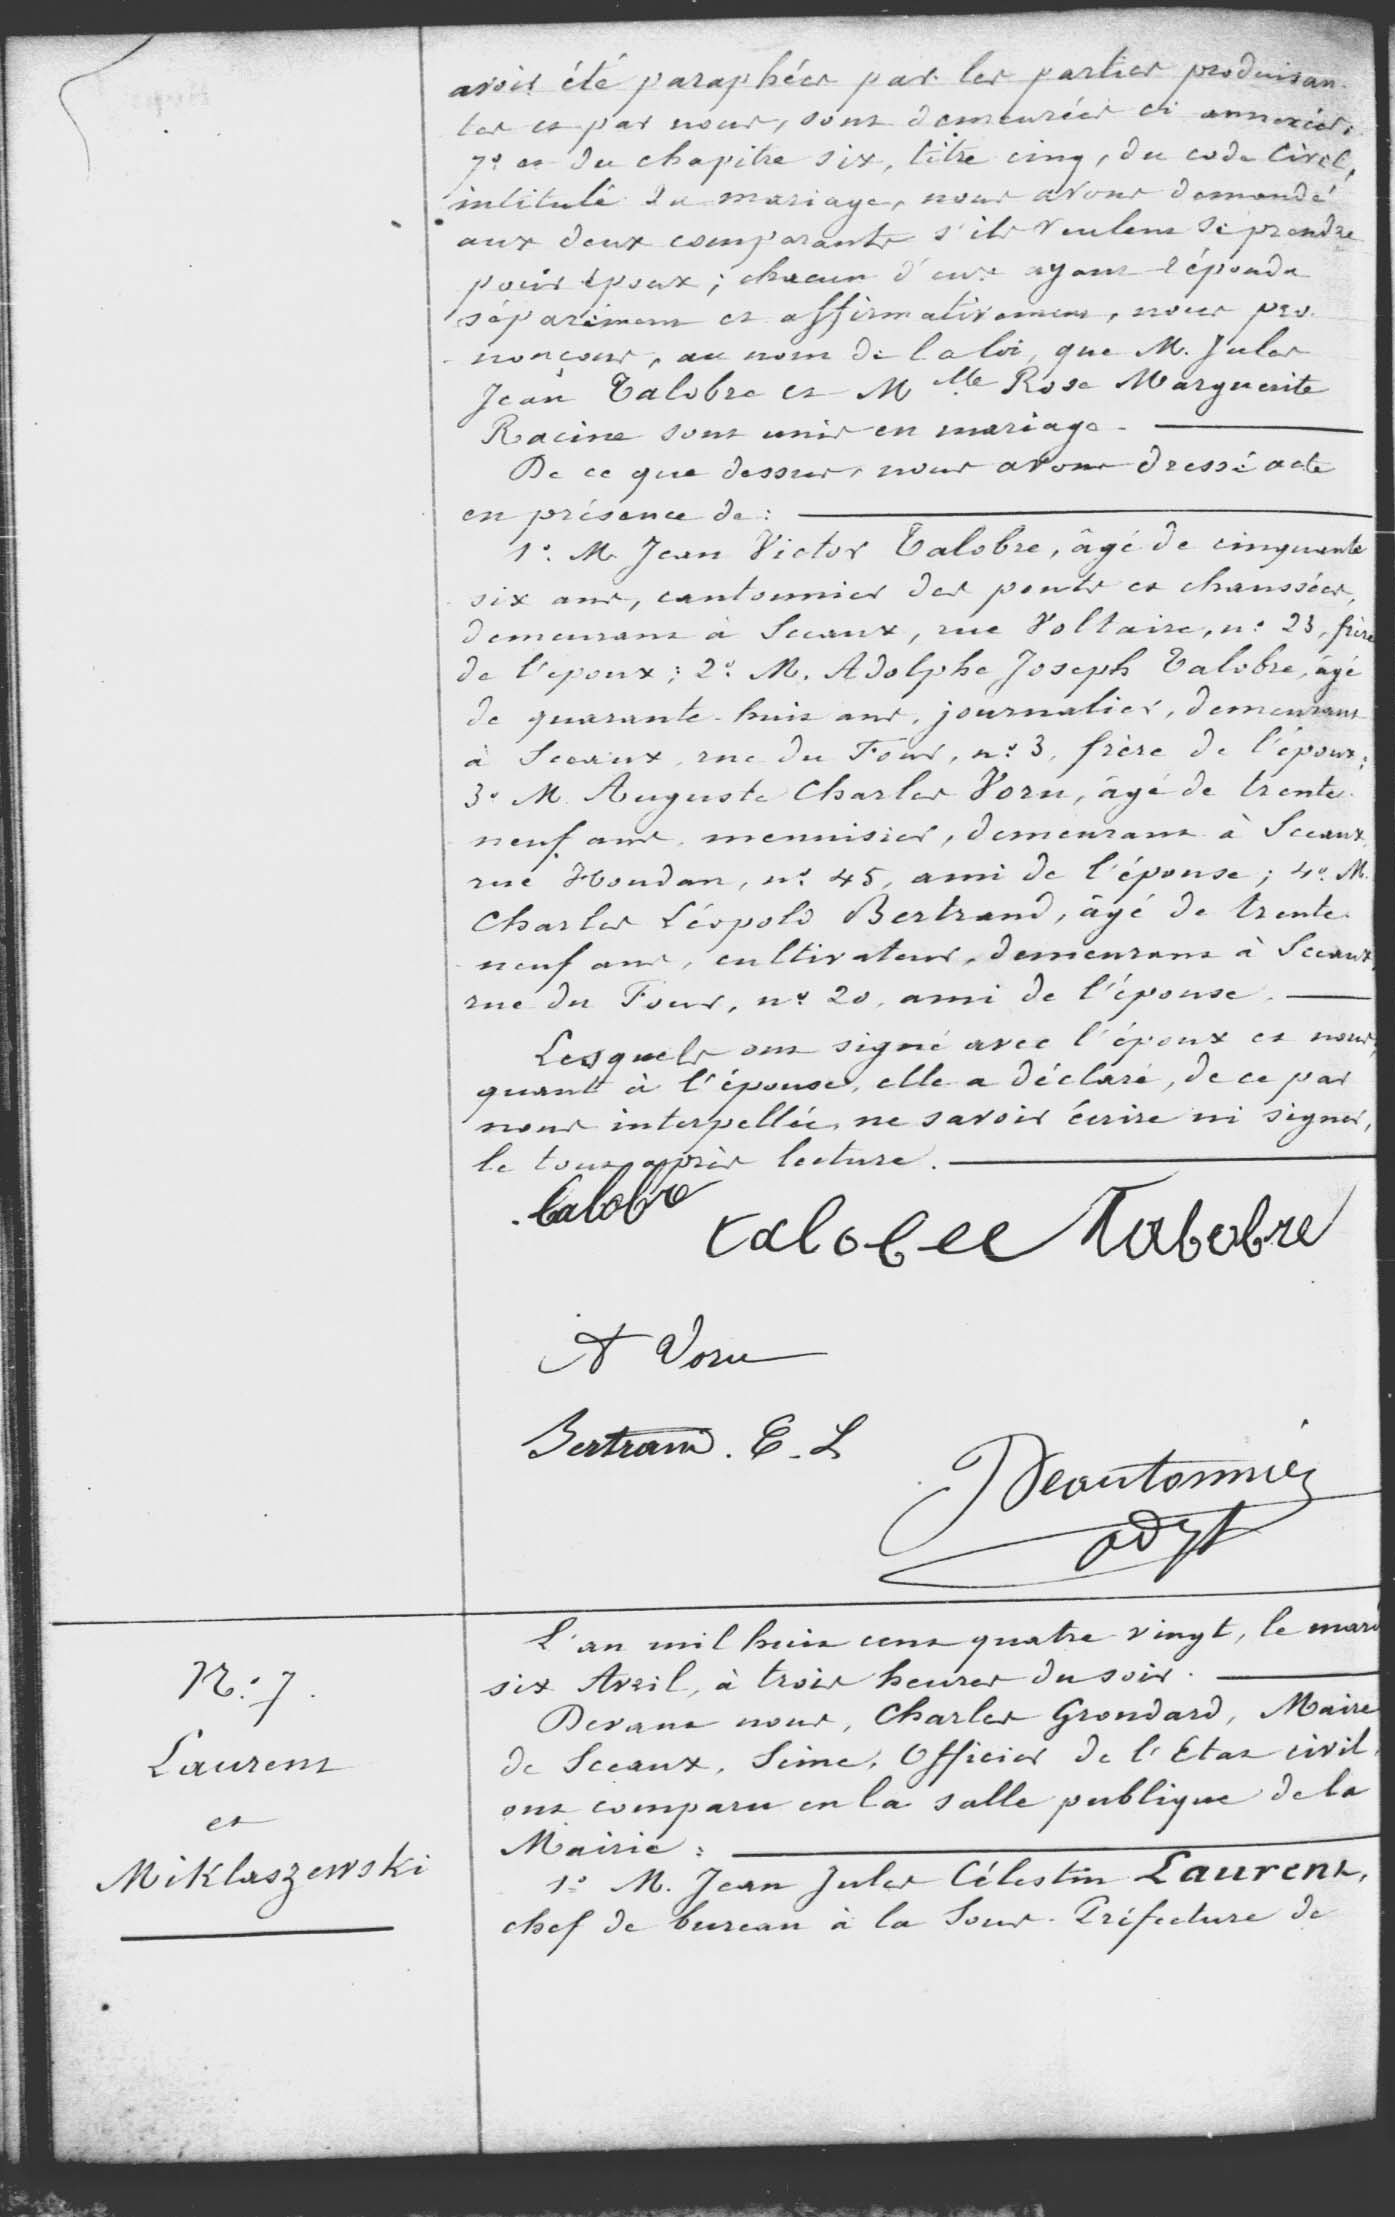avoir été paraphées par les parties produisan
tot ce par nous, dans demeurées et annexées,
7° du chapitre six, titre cinq, du code civil,
intitulé du mariage, nous avons demandé
aux deux comparants s'ils veulent se prendre
pour époux; chacun d'eux ayant répondu
séparément et affirmativement, nous pro
nonçons, au nom de la loi que M. Jules
Jean Talbro et Mlle Rosa Marguerite
Racine sont unis en mariage. -
De ce que dessus nous avons dressé acte
en présence de: -
1° M Jean Victor Calobre, âgé de cinquante
six ans, cantonnier des ponts et chaussées
demeurant à Sceaux, rue Voltaire, n° 23, frère
de l'époux; 2° M. Adolphe Joseph Talobre, âgé
de quarante huit ans, journalier, demeurant
à Sceaux, rue du Four, n°3 frère de l'époux;
3° M Auguste Charles Vorn, âgé de trente
neuf ans, menuisier, demeurant à Sceaux
rue Houdan, n° 45, ami de l'épouse; 4° M
Charles Léopold Bertrand âgé de trente
neuf ans, en cultivateur, demeurant à Sceaux
rue du Four, n° 20, ami de l'épouse. -
Lesquels ont signé avec l'époux et nous
quant à l'épouse elle a déclaré, de ce par
nous interpellée, ne savoir écrire ni signer,
le tout après lecture. -
N° 7
Lauren
et
Miklaszewski
L'an mil huit cent quatre-vingt, le mard
six Avril, à trois heures du soir -
Devant nous, Charles Grondard, Maire
de Sceaux, Seine, Officier de l'Etat civil,
ont comparu en la salle publique de la
Mairie: -
1° M. Jean Jules Célestin Laurent
chef de bureau) la Sous Préfecture de
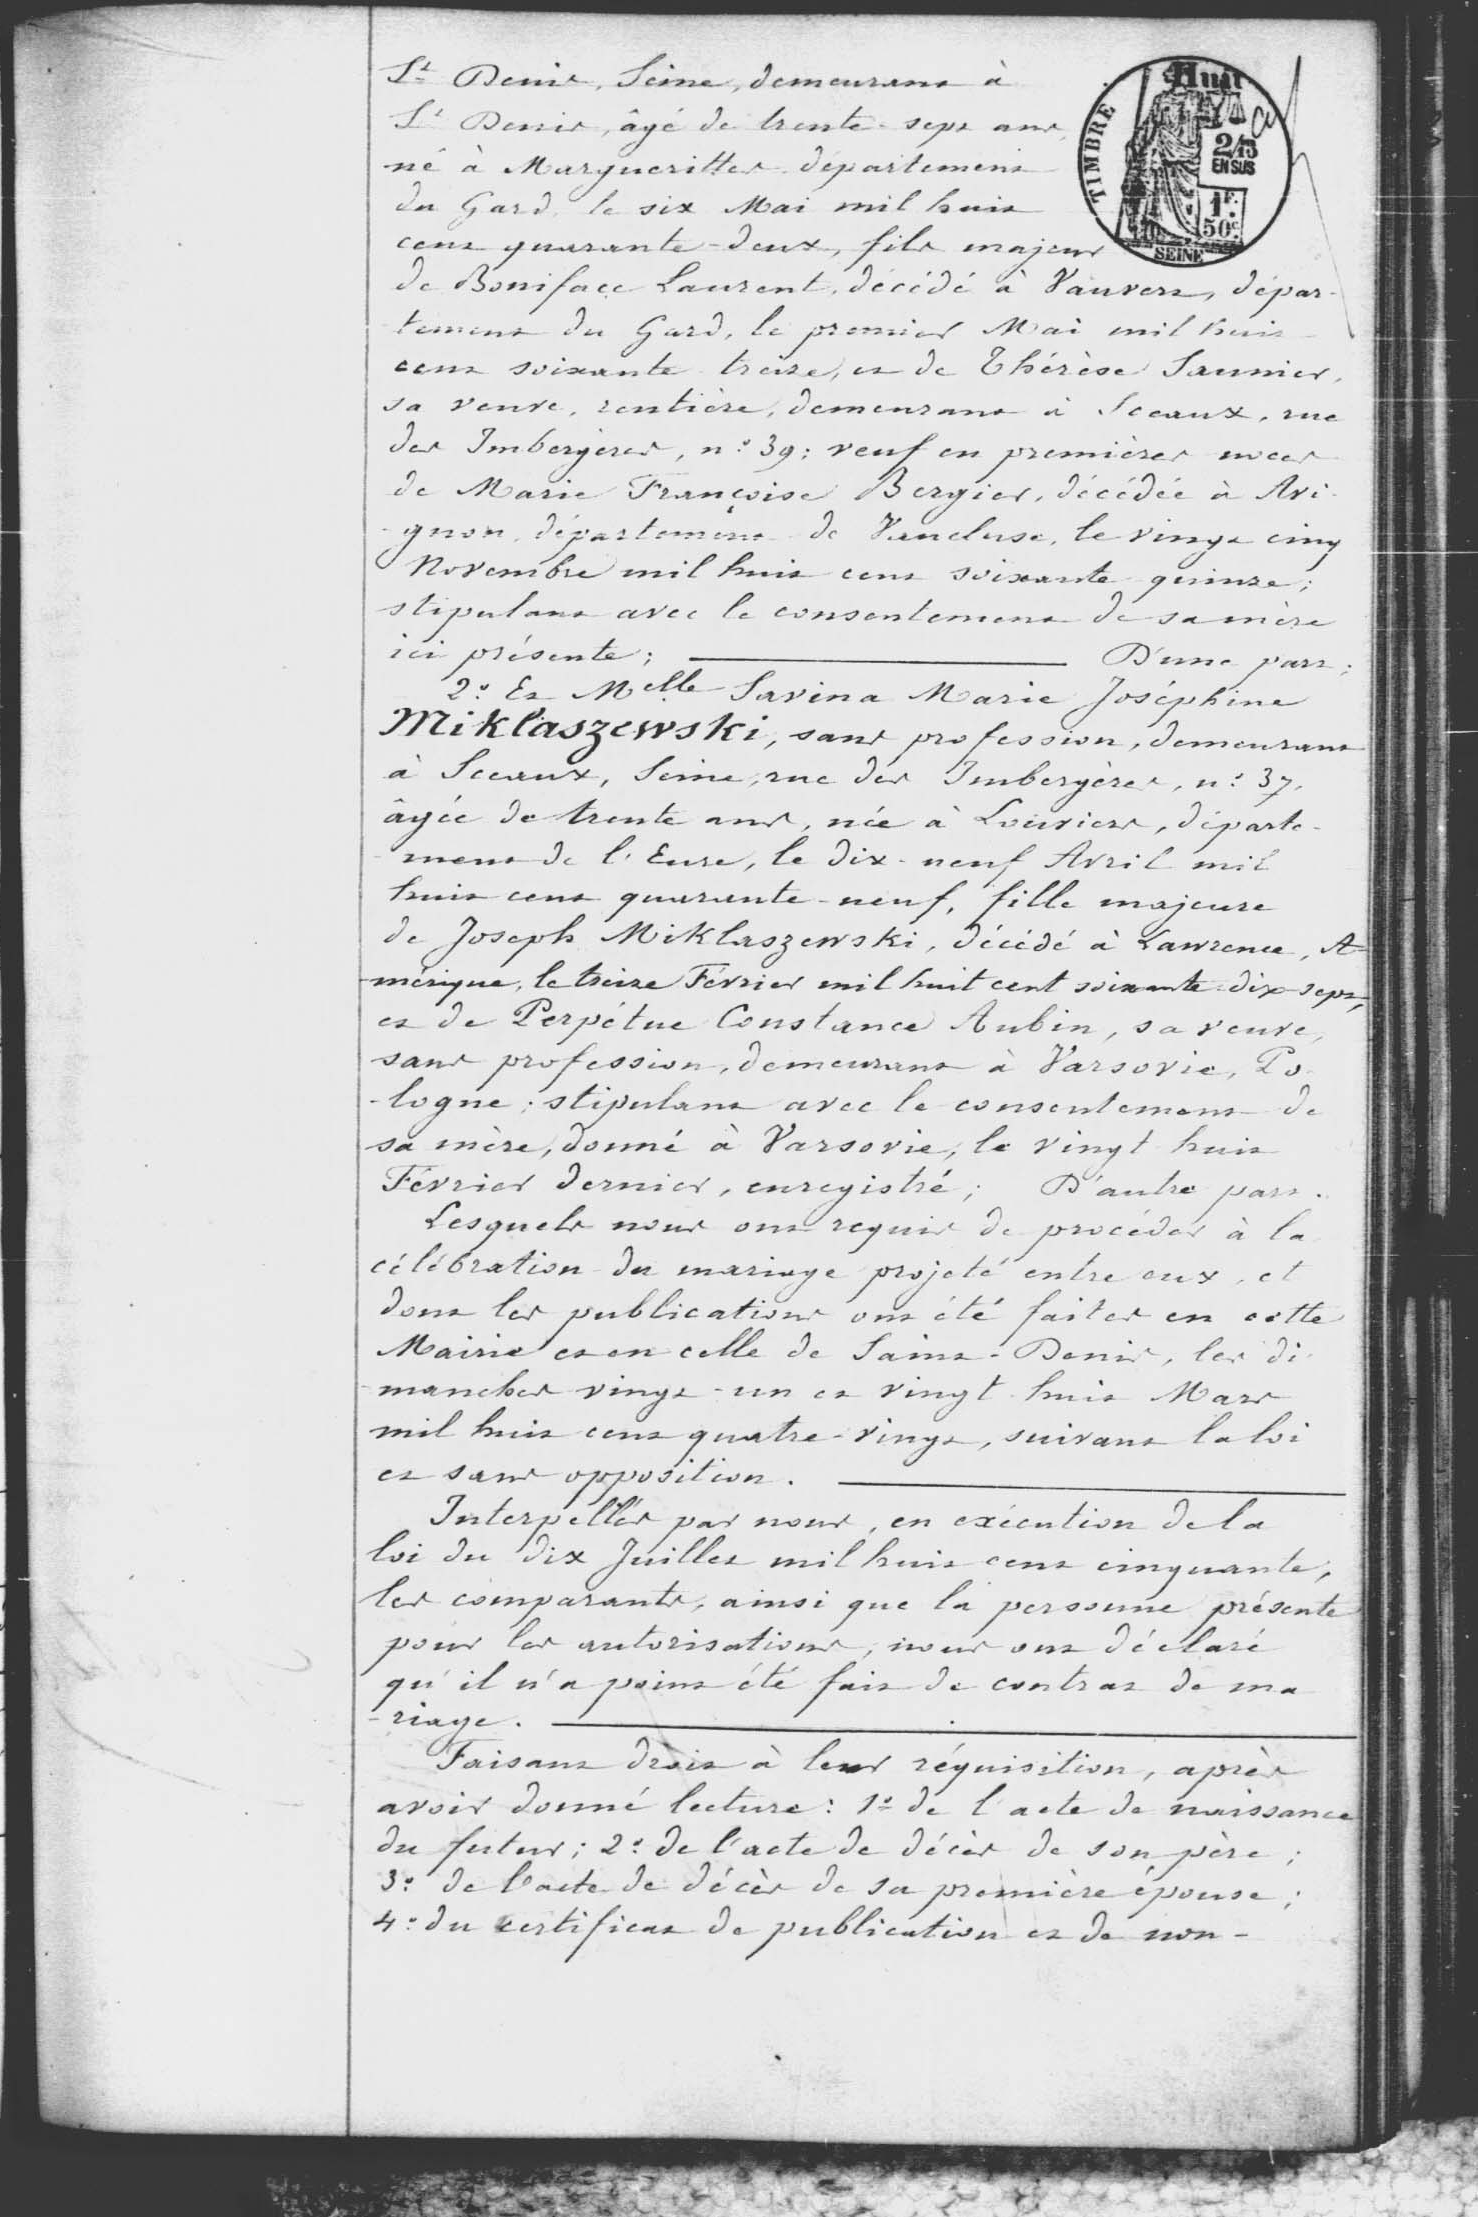Saint Denis, Seine, demeurant à
Saint Denis âgé de trente-sept ans
né à Margueritte département
du Gard le six mai mil huit
cent quarante-deux, fils majeur
de Boniface Laurent, décédé à Vauvere, dépar-
tement du Gard, le premier mai mil huit
cent soixante trois et de Thérèse Saunier,
sa veuve, rentière, demeurant à Sceaux, rue
des Imbergères, n°39: veuf en premières noces
de Marie Françoise Bergier, décédée à Avi-
gnon, département de Vaucluse, le vingt-cinq
Novembre mil huit cent soixante-quinze
stipulant avec le consentement de sa mère
ici présente; -D'une part;
2° Et Melle Savina Marie Joséphine
Miklaszewski, sans profession, demeurant
à Sceaux, Seine, rue des Imbergère, n° 37
âgée de trente ans, née à Lounier, départe-
ment de l'Eure, le dix-neuf Avril mil
huit cent quarante-neuf, fille majeure
de Joseph Miklaszwski, décédé à Lawrence, A-
mérique, le treize Février mil huit cent soixante dix-sept,
et de Perpétue Constance Aubin, sa veuve,
sans profession, demeurant à Varsovie, Po-
-logne: stipulant avec le consentement de
sa mère, donné à Varsovie, le vingt huit
Février dernier, enregistré; D'autre part.
Lesquels nous ont requis de procéder à la
célébration du mariage projeté entre eux, et
dont les publications ont été faites en cette
Mairie et en celle de Saint-Denis, les di
manches vingt-un et vingt huit Mars
mil huit cent quatre vingt, suivant la loi
et sans opposition. -
Interpellés par nous, en exécution de la
loi du dix Juillet mil huit cent cinquante;
les comparants, ainsi que la personne présente
pour les autorisations, nous ont déclaré
qu'il n'a point été fais de contrat de ma
-riage. -
Faisant désir à leur réquisition, après
avoir donné lecture: 1° de l'acte de naissance
du futur; 2° de l'acte de décès de son père;
3° de l'acte de décès de sa première épouse;
4° du certificat de publication en le non